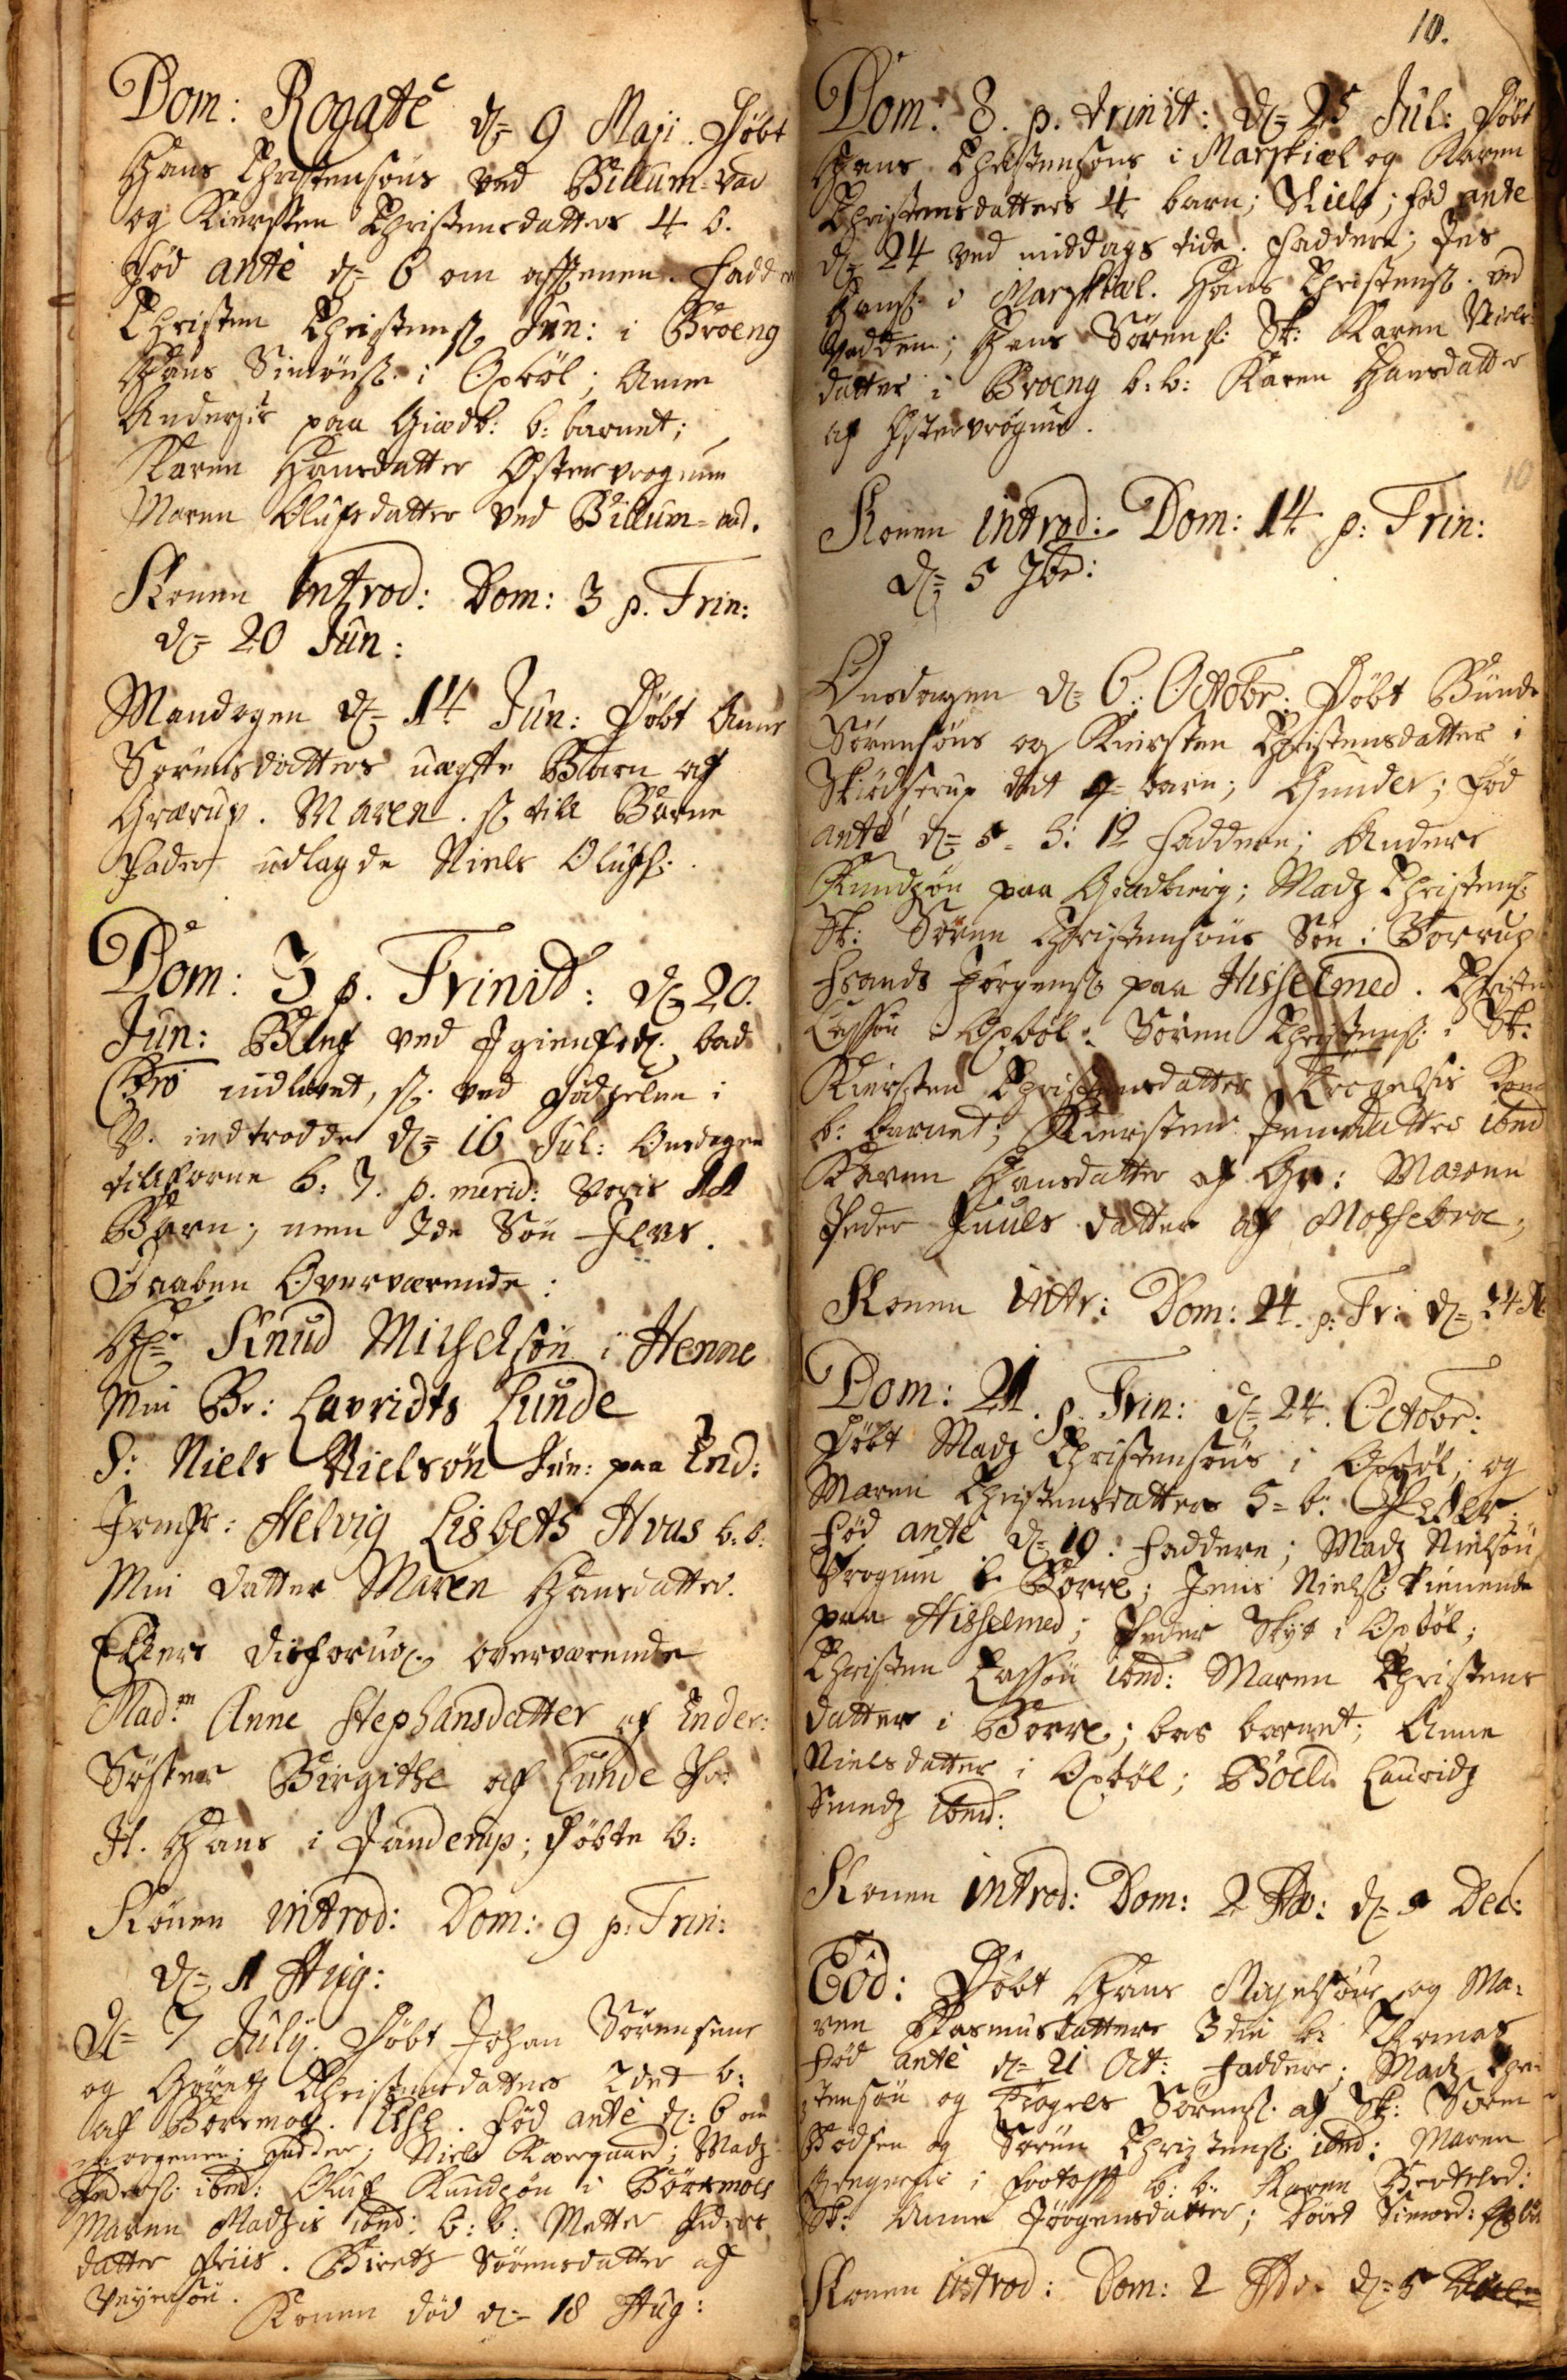Dom: Rogate d= 9  Maji Døbt
Hans Christensøns ved Billum=vad
og Kiersten Christensdatters 4 b.
fød ante d= 6 om afftenen. Faddere
Christen Christens Jun: i Broeng
Hans Simons. i Oxbøl; Anne
Andersis paa Giædb: b: barnet;
Karen Hansdatter Østervrogum
Maren Olufsdatter ved Billum=vad.
Konen introd: Dom. 3 p.Trin:
d= 20 Jun:
Mandagen d=.14 Jun: Døbt Anne
Sørensdatters uægte Barn af
Grærup. Maren. s till Barne
fader udlagde Niels Olufs.
Dom: 3 p. Trinit: d= 20.
Jun: Blef ved Igienfod. bad
##o indla#et, s. ved fødselen i
## indtrodde d= 16 JuL. Onsdagen
tillforne ##: 7. p. merid: voris 11
Barn; men 7de Søn Jens.
Daaben Overværende:
Hr Knud Michelsøn i Henne
min Br: Lavridtz Lunde,
S: Niels Nielsøn Jun: paa End:
Jomfr: Helvig Lisbeth Hvas b. B:
Min datter Maren Hansdatter.
Ellers desforud overværende
Madm Anne Stephansdatter af Ender:
Søster Birgithe af Lunde Pr:
H. Hans i Janderup; Døbte B.
Konen introd: Dom: 9 p: Trin:
d.1.Aug:
D. 7 Julij Døbt Johan Sørensens
og Gøreth Christensdatters 2det b:
af Borsmoes. Else. fød ante d: 6 om
morgenen; Faddere Niels Kæregaard; Madz
Peders ibm: Oluf Knudsøn i Børsmoes
Maren Madtsis ibm: b: b: Mette Peders
datter Friis. Bireth Sørensdatter af
Veyersøe.
Konen død d= 18 Aug:
10
10
Dom. 8. p. Trinit: d= 25 Jul: Døbt
Hans Christensøns i Marskiæl og Karen
Christensdatters 4 barn; Niels; fød ante
d 24 ved middags tide. Faddere; Jes
Hans i Marskiæl. Hans Christens. ved
Vadden; Hans Sørens: Sk: Karen Niels¬
datter i Broeng b:  b: Karen Hansdatter
af Østervrøgum.
Konen introd: Dom: 14. p: Trin:
d= 5 Sbr:
Onsdagen d= 6: Octobr: Døbt Bunde
Sørensøns og Kiersten Christensdatters i
Skiødstrup det 4= barn; Gunder; fød
ante d= 5= S: 12 Faddere; Anders
Knudzøn paa Giædbierg, Madz Christens
Sk: Søren Christensøns Søn i Borrup
Frands Jørgens paa Hisselmed. Christen
Lassøn i Oxbøl: Søren Christens i Sk:
Kiersten Christensdatter Trogelsis Kone
b: barnet; Kiersten Jensdatter ibm
Karen Hansdatter af Gr: Maren
Peder Juuls datter af Moesebroe;
Konen intr. Dom: 24 p: Tr: d= 24 N.
Dom.21. p. Trin: d= 24 Octobr:
Døbt Madz Christensøns i Oxbøl og
Maren Christensdatters 5= b. Peder
fød ante d= 10. Faddere; Madz Nielsøn
Vrogum i Borre; Jens Nielsen tienende
paa Hisselmed; Peder Skyt i Oxbøl;
Christen Lassøn ibm: Maren Christens
datter i Borre; bar barnet; Anne
Nielsdatter i Oxbøl; Boeld Lauridz
Smedz ibm:
Konen introd: Dom: 2 Adv. d= 5 Dec:
Eod: Døbt Hans Michelsøns og Ma¬
ren Rasmusdatters 3die b. Thomas
fød ante d= 21 Oct: Faddere; Madz Chri
stensøn og Trogels Sørensn af Sk: Soren
Bodsen og Søren Christens ibm: Maren
Gregersis i Frotofft b: b: Karen Bertelsd:
Sk: Anne Jørgensdatter; Doret Simonsd: Oxbøl.
Konen introd: Dom: 2 Adv. d= 5 Dec.
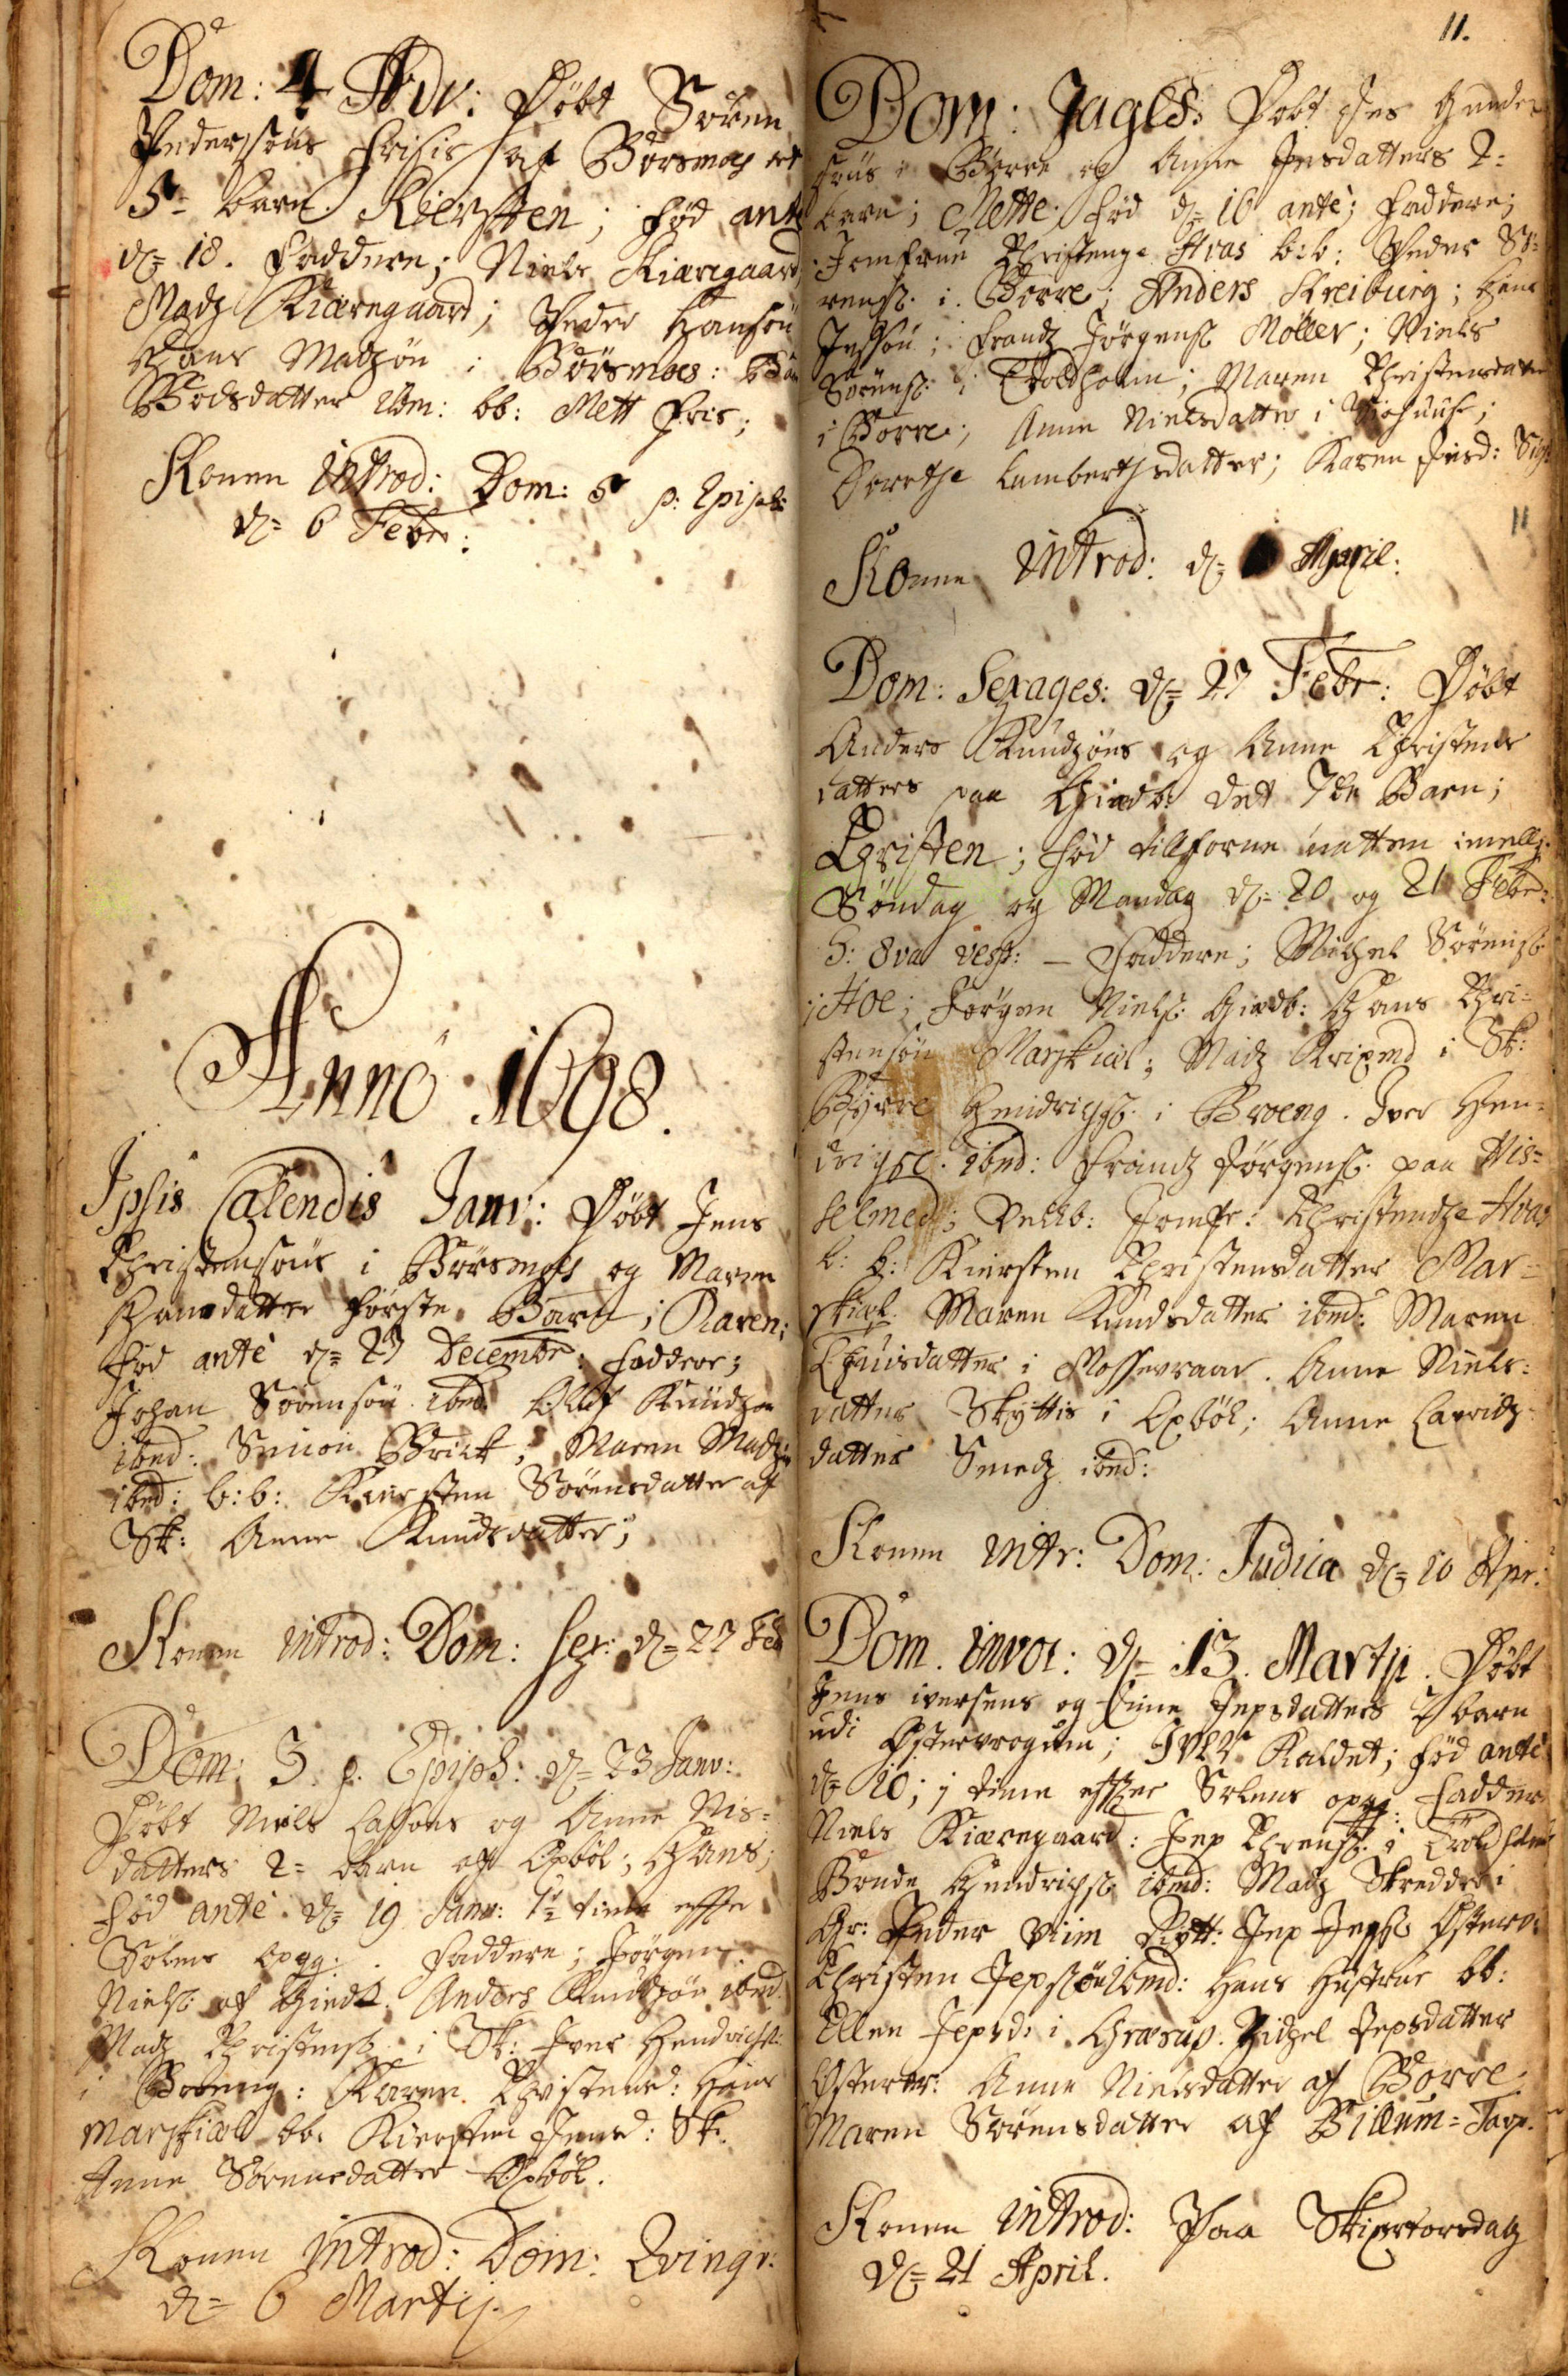Dom:4 Adv: Døbt Søren
Pedersøns Frisis af Borsmoes det
5= Barn. Kiersten; fød ante
d= 18. Faddere; Niels Kiæregaard,
Madz Kiæregaard, Peder Hansøn,
Hans Madzøn i Børsmoes: ##
Bodsdatter ibm: bb:, Mett Fris;
Konen introd: Dom: 5 p: Epiph:
d= 6 Febr:
Anno 1698.
Ipsis Calendis Janv: Døbt Jens
Christensøns i Børsmos og Maren
Hansdatters første Barn; Karen;
fød ante d= 27 Decembr: Faddere;
Johan Sørensøn ibm. Oluf Knudzon
ibm: Simon Brick, Maren Madzis
ibm b: b: Kiersten Sørensdatter af
Sk; Anne Knudzdatter;
Konen introd: Dom: Sex: d= 27 Feb
Dom: 3. p. Epiph. d= 23 Janv:
Døbt Niels Lassøns og Anne Nis¬
datters 2= barn af Oxbøl; Hans;
fød ante d= 19 Janv: 1½ time effter
Solens Opgg: Faddere; Jørgen
Niels af Giedb., Anders Knudzøn ibm.
Madz Christens i Sk: Jens Hendichs
i Broeng: Karen Christensd: Hans
Marskiæl bb. Kiersten Jensd: Sk
Anne Sørensdatter Oxbøl.
Konen introd: Dom: Qvinqv.
d= 6 Martij.
11
11
Dom: 7ages. Døbt Jes Gundes
søns i Borre og Anne Jesdatters 2=
barn; Mette fød d= 16 ante; Faddere;
Jomfrue Christenze Hvas b: b: Peder Sø¬
rens i Borre; Anders Kreiberg; Hans
Jessøn; Frandz Jørgens Møller; Niels
Sørens i Troldholm; Maren Christensdatter
i Borre; Anne Nielsdatter i Siohuuse;
Dorethe L##mbertsdatter; Karen Jesd. S##
Konen introd: d.  ## April:
Dom: Sexages: d= 27 Febr: Døbt
Anders Knudzøns og Anne Christens
datters paa Giædb: det 7de Barn;
Christen; fød tillforne natten imell:
Søndag og Mandag d= 20 og 21 Febr:
S: 8va vesp: - Faddere; Michel Sørens
i Hoe; Jørgen Niels Giædb: Hans Chri¬
stensøn i Marskiæl; Madz Krixmd i Sk:
Byrre Hendichs i Broeng. Iver Hen¬
drichs ibm: Frandz Jørgens paa His¬
selmed; Vellb: Jomfr: Christenze Hvas
b: B: Kiersten Christensdatter Mar¬
skiæl. Maren Knudsdatter ibm: Maren
Thuisdatter i Mossevraae. Anne Niels¬
datter Skyttis i Oxbøl; Anne Lavridtz¬
datter Smedz ibm:
Konen intr: Dom: Judica d= 10 Apr:
Dom. Invoc: d= 13. Martji Døbt
Jens iersens og Anne Jepsdatters 2 barn
udi Østervrogum; Iver kaldet; fød ante
d= 10; 1 time effter Solens opgg: Faddere
Niels Kiæregaard: Jep Christens i Troldholm
Bonde Hendrichs ibm: Madz Skredder i
Gr. Peder Viim Rytt: Jep Jeps Østerv:
Christen Jepsøn ibmd: Hans Hustrue bb:
Ellen Jepsd. i Grærup. Zidzel Jepsdatter
Østervr: Anne Nielsdatter af Borre,
Maren Sørensdatter af Billum=Tavp.
Konen introd: Paa Skiertorsdag
d= 21 April.